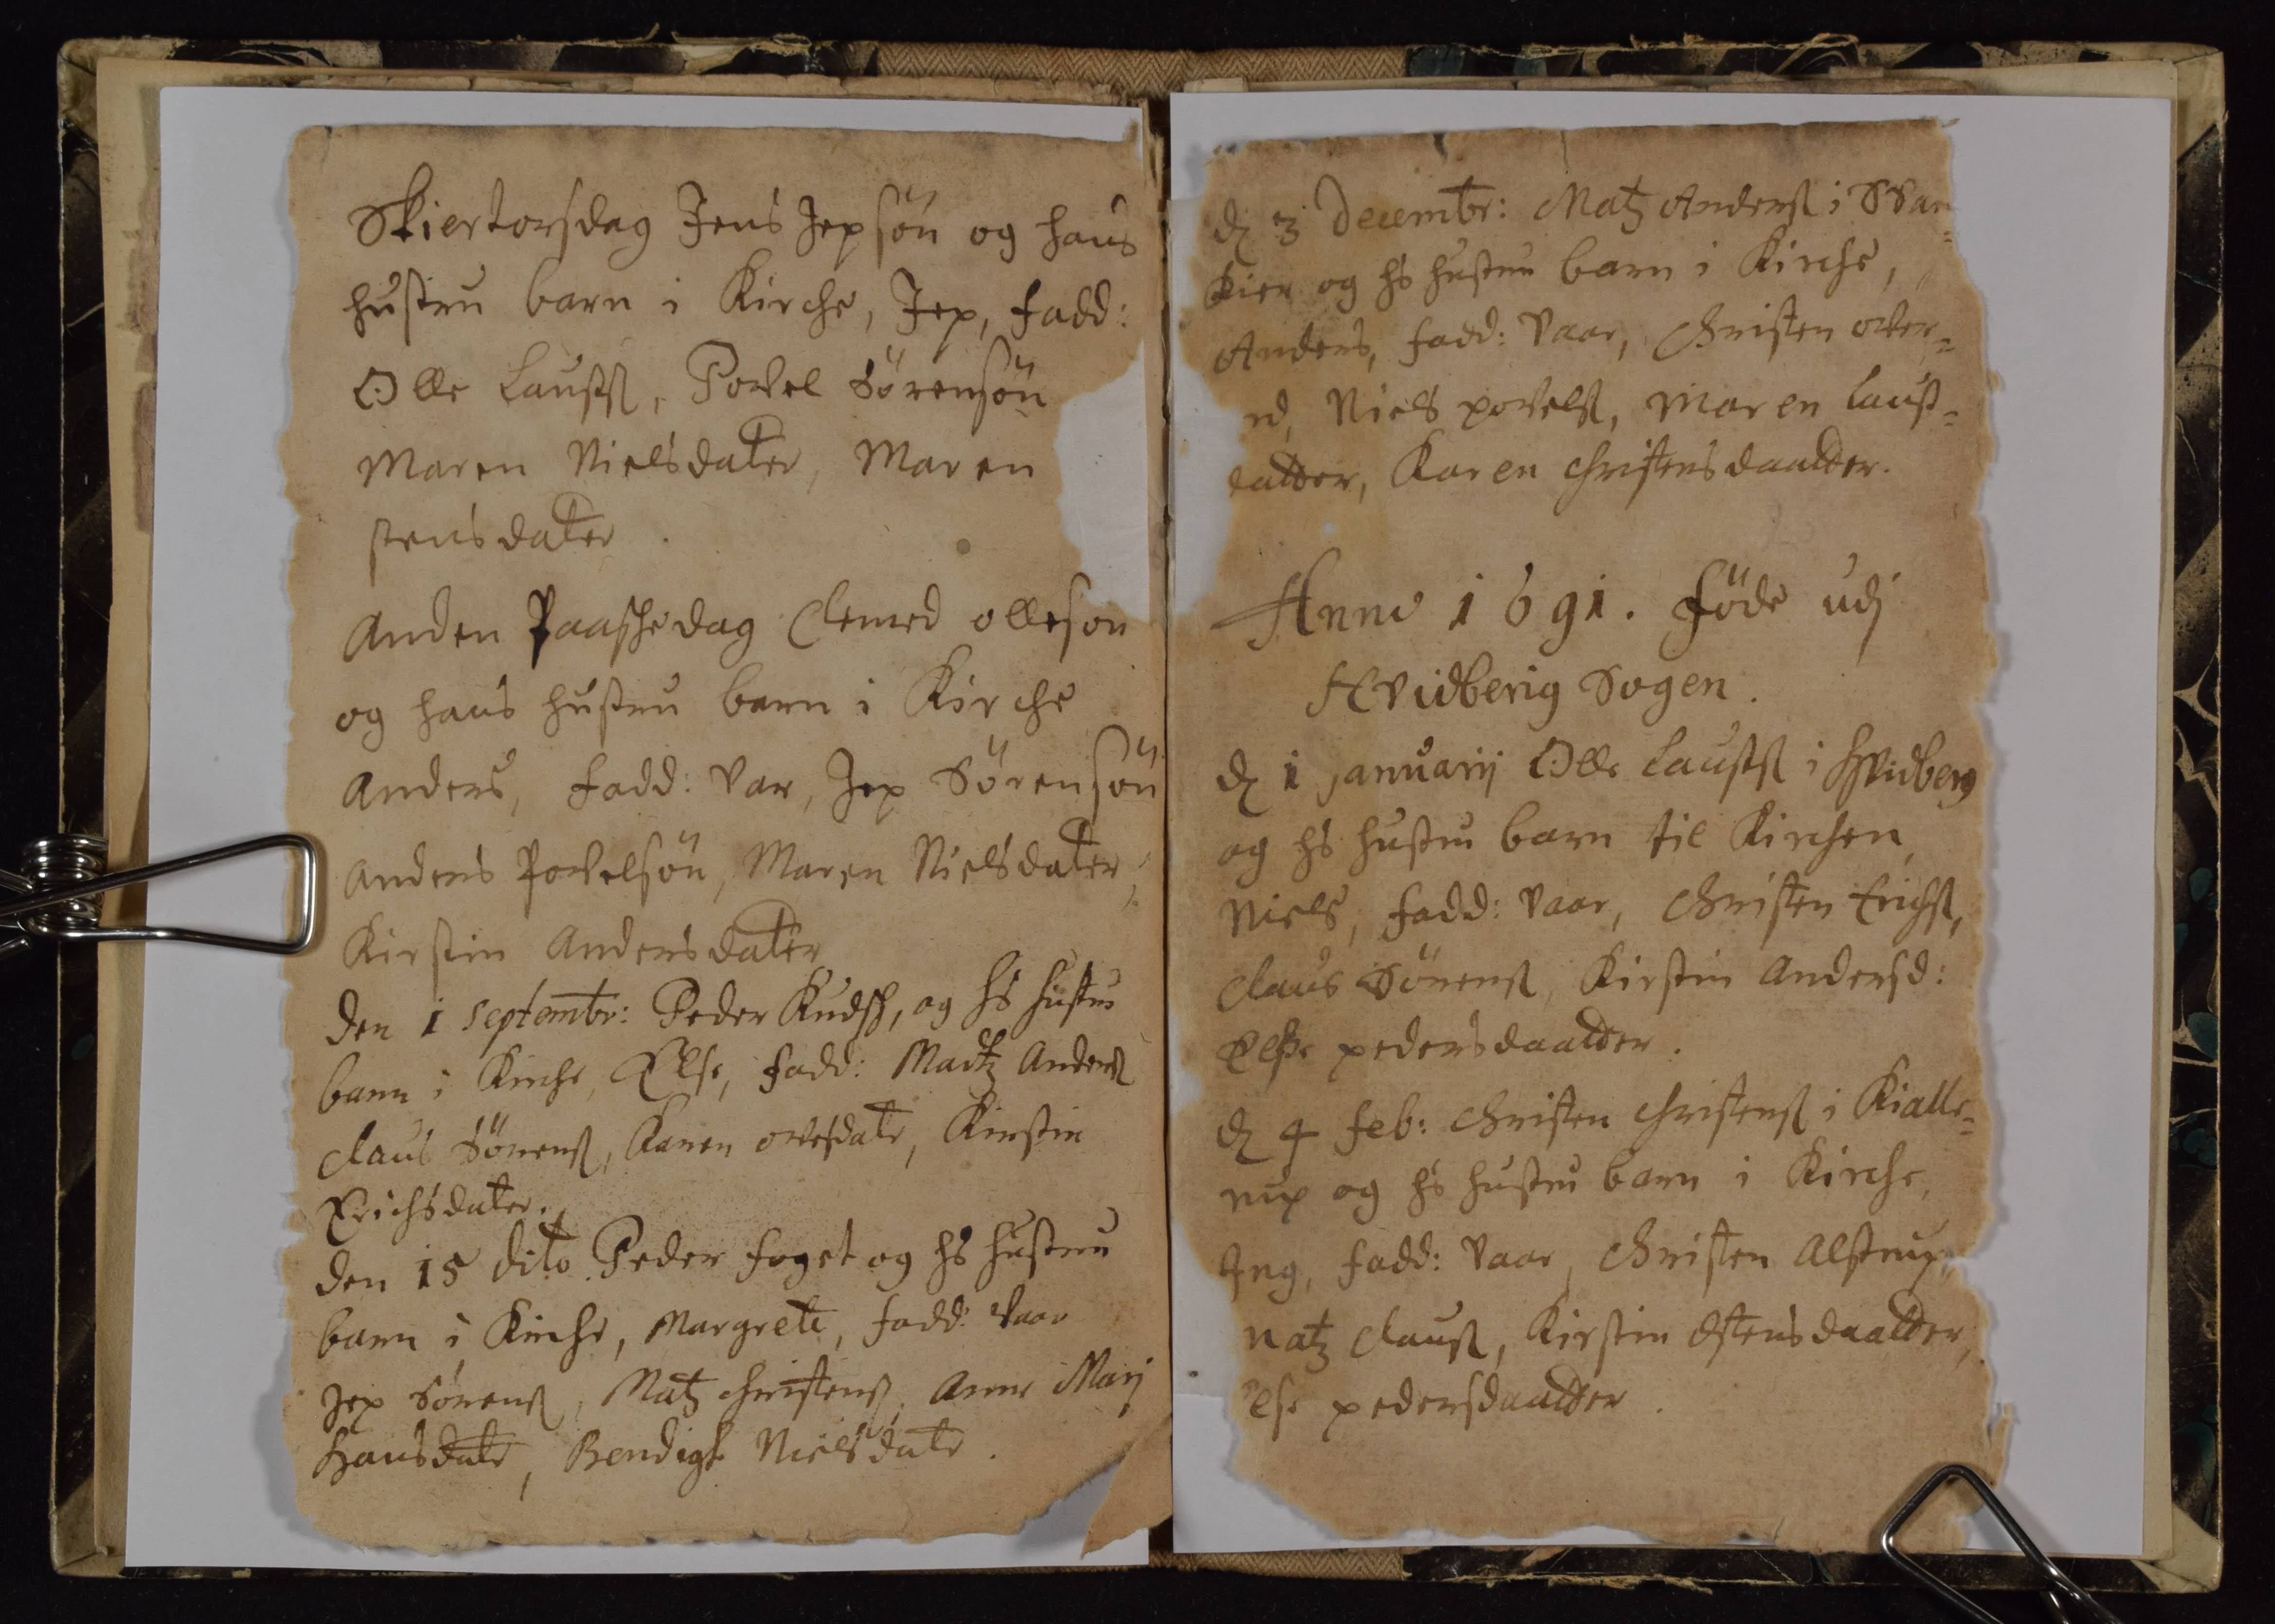Skiertorsdag Jens Jepsøn og hans
hustru barn i Kirche, Jep Fadd:
Olle Lausts, Povel Sørensøn
Maren Nielsdater, Maren
stensdater
Anden Paashedag Clemed Olleson
og hans hustru barn i Kirche
Anders, Fadd: var Jep Sørensøn
Anders Povelsøn, Maren Nielsdater
Kirstin Andersdater
Den 1 Septembr: Peder Kudh, og hs hustru
barn i Kirche, Else, Fadd: Madtz Anders
Claus Sørens, Karen Ovesdatr Kirstin
Erichsdater.
den 15 dito Peder foget og hs hustru
barn i Kiiche, Margrete, Fadd: vaar
Jep Sørens, Matz Christens, Anne Marj
Hansdatr Bendigt Nielsdatr
d 3 Decembr: Matz Anders i Svan
kier og hs hustru barn i Kirche,
Anders Fadd: vaar Christen over¬
rd, Niels povels, Maren Laust¬
datter, Karen Christensdaatter.
Anno 1691. Føde udj
Hvidberig Sogen
d. 1 Januarij Olle Lausts i Hvidberg
og hs hustru barn til Kirchen,
Niels, Fadd: vaar, Christen Erichs,
Claus Sørens, Kirstin Andersd:
Else pedersdaatter
d 4. Feb: Christen Christens i Kialle¬
rup og hs hustru barn i Kirche
Ing, Fadd: vaar, Christen Alstrup
natz Claus Kirstin Ostensdaatter
Else pedersdaatter
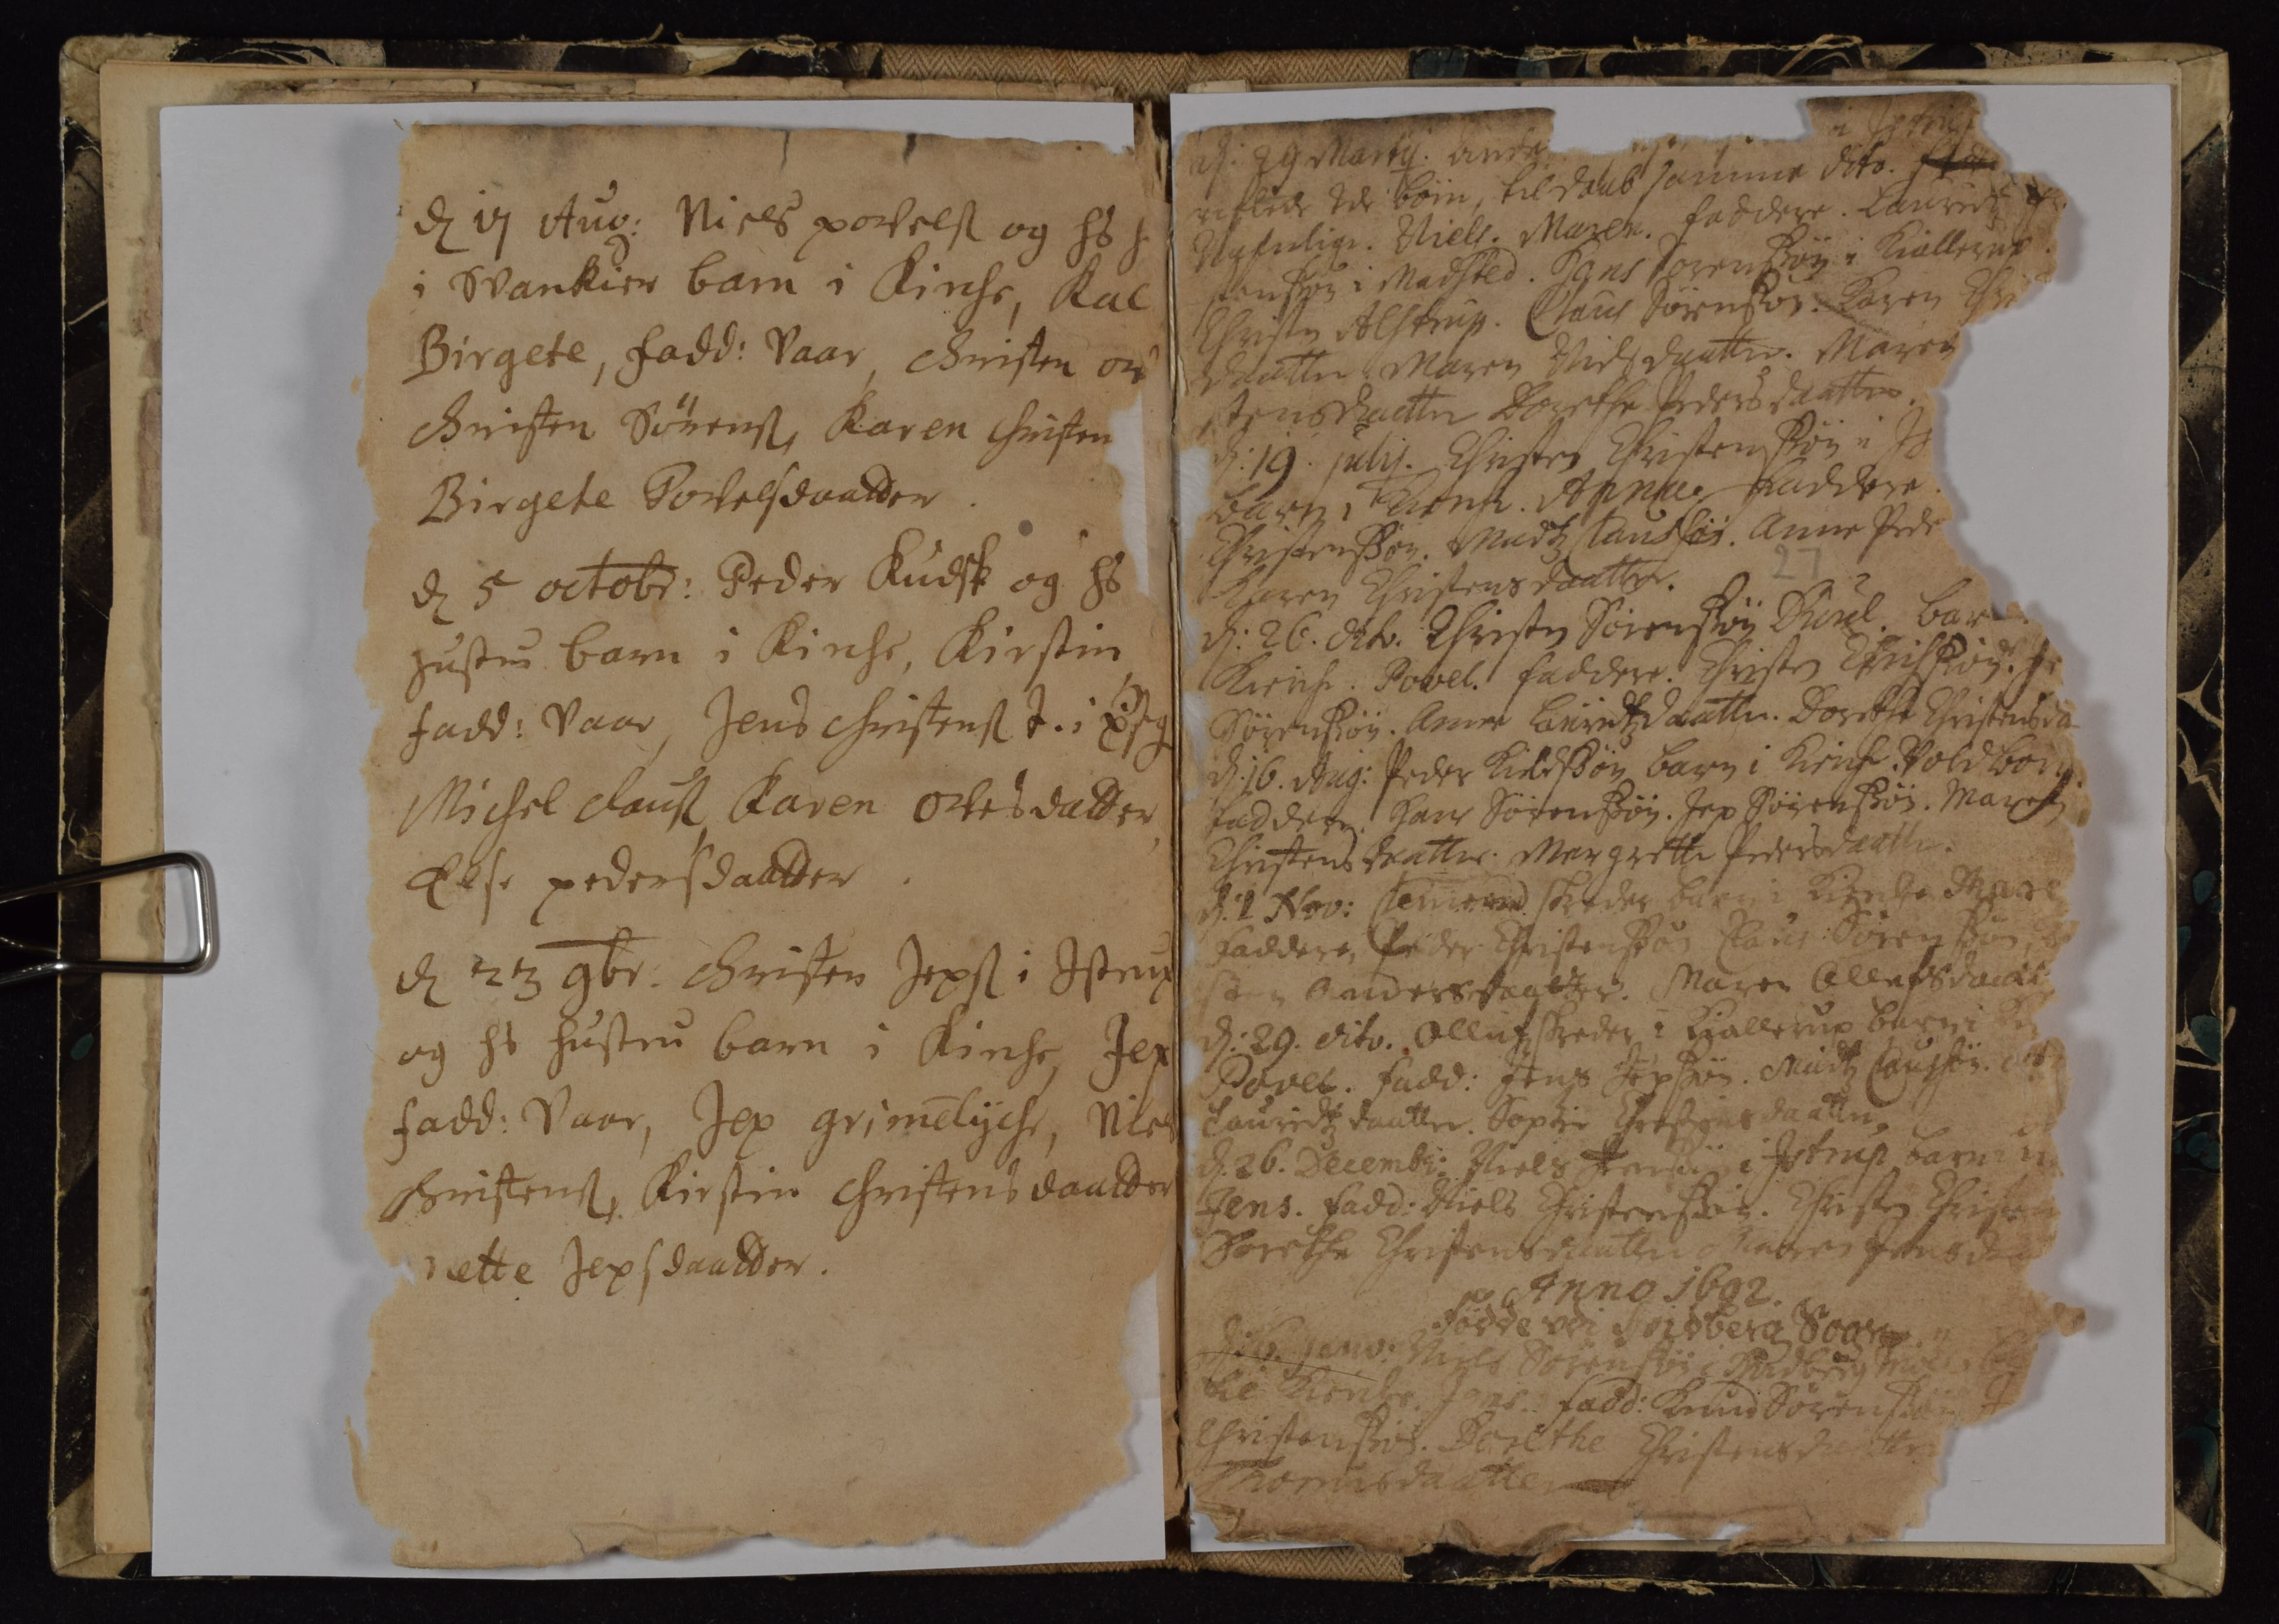d 17 Aug: Niels povels og hs ##
i Svankier barn i Kirhe, Kal
Birgete, Fadd: vaar Christen Ov
christen Sørens, Karen Christen
Birgete Povelsdaatter
d 5 octobr: Peder Kudsk og hs
hustru barn i Kirche, Kirstin
Fadd: vaar, Jens Christens ## i ##
Michel Claus Karen Ovesdatter
Else pedersdaatter
d 23 9br, Christen Jeps i Istrup
og hs hustru barn i Kirche, Jep
Fadd: vaar, Jep Grimmelyche, Nie## 
Christens, Kirstin Christensdaatter
Mætte Jepsdaatter
dd: 29 Martij. Ande
i Istrup
##lede 2de børn til daab samme dito ##
Nafnlige. Niels. Maren. Faddere Lauridtz ##
stenssen i Madsted. Hans Sørenssøn i Kiallerup
Christen Alstrup. Claus Sørenssøn Karen Chri
daatter Maren Nielsdaatter. Maren
stensdaater. Dorethe Pedersdaatter
d: 19 Julij. Chisten Christenssøn i Is
barn i Kirche, Anne Faddere
Christenssøn, MadtzClaussøn. Anne Pede  
Karen Christensdaatter.
d: 26 Ocbr. Christen Sørenszøn Du##l barn
Kirche. Povel. Faddere. Christen Erichssøn. ##
Sørenssøn Anne Lauridtzdaatter. Dorethe Christensda
d: 16. Aug: Peder Kieldssøn barn i Kirche Voldborg
Faddere. Hans Sørenssøn, Jep Sørenssøn. Maren
Christensdaatter. Margrette Pedersdaatter.
d. 1 Nov: Clemend skreder barn i Kirche ##
Faddere Peder Christenssøn Claus Sørenssøn,   
sten Andersdaatter. Maren Ollefsdaatt
d: 29 dito. Olluf skreder i Kiallerup barn i
Povel. Fadd: Jens Jepssøn. Madtz Claustsøn. och
Lauridtz daatter. Sophi Christens daatter
d 26: Decembr: Niels Jenssøn i Istrup barn i
Jens Fadd. Niels Christenssøn. Christen Christen
Dorethe Christensdatter Maren Jensda  
Anno 1692.
Fødde vdi Hvidberg Sogen
d: 6. Janv: Niels Sørenssøn Hvidberg Mølle barn
til Kierhe Jens. Fadd: Knud Sørenssøn
Christenssøn. Dorethe Christensdaatter
Thomsdatter